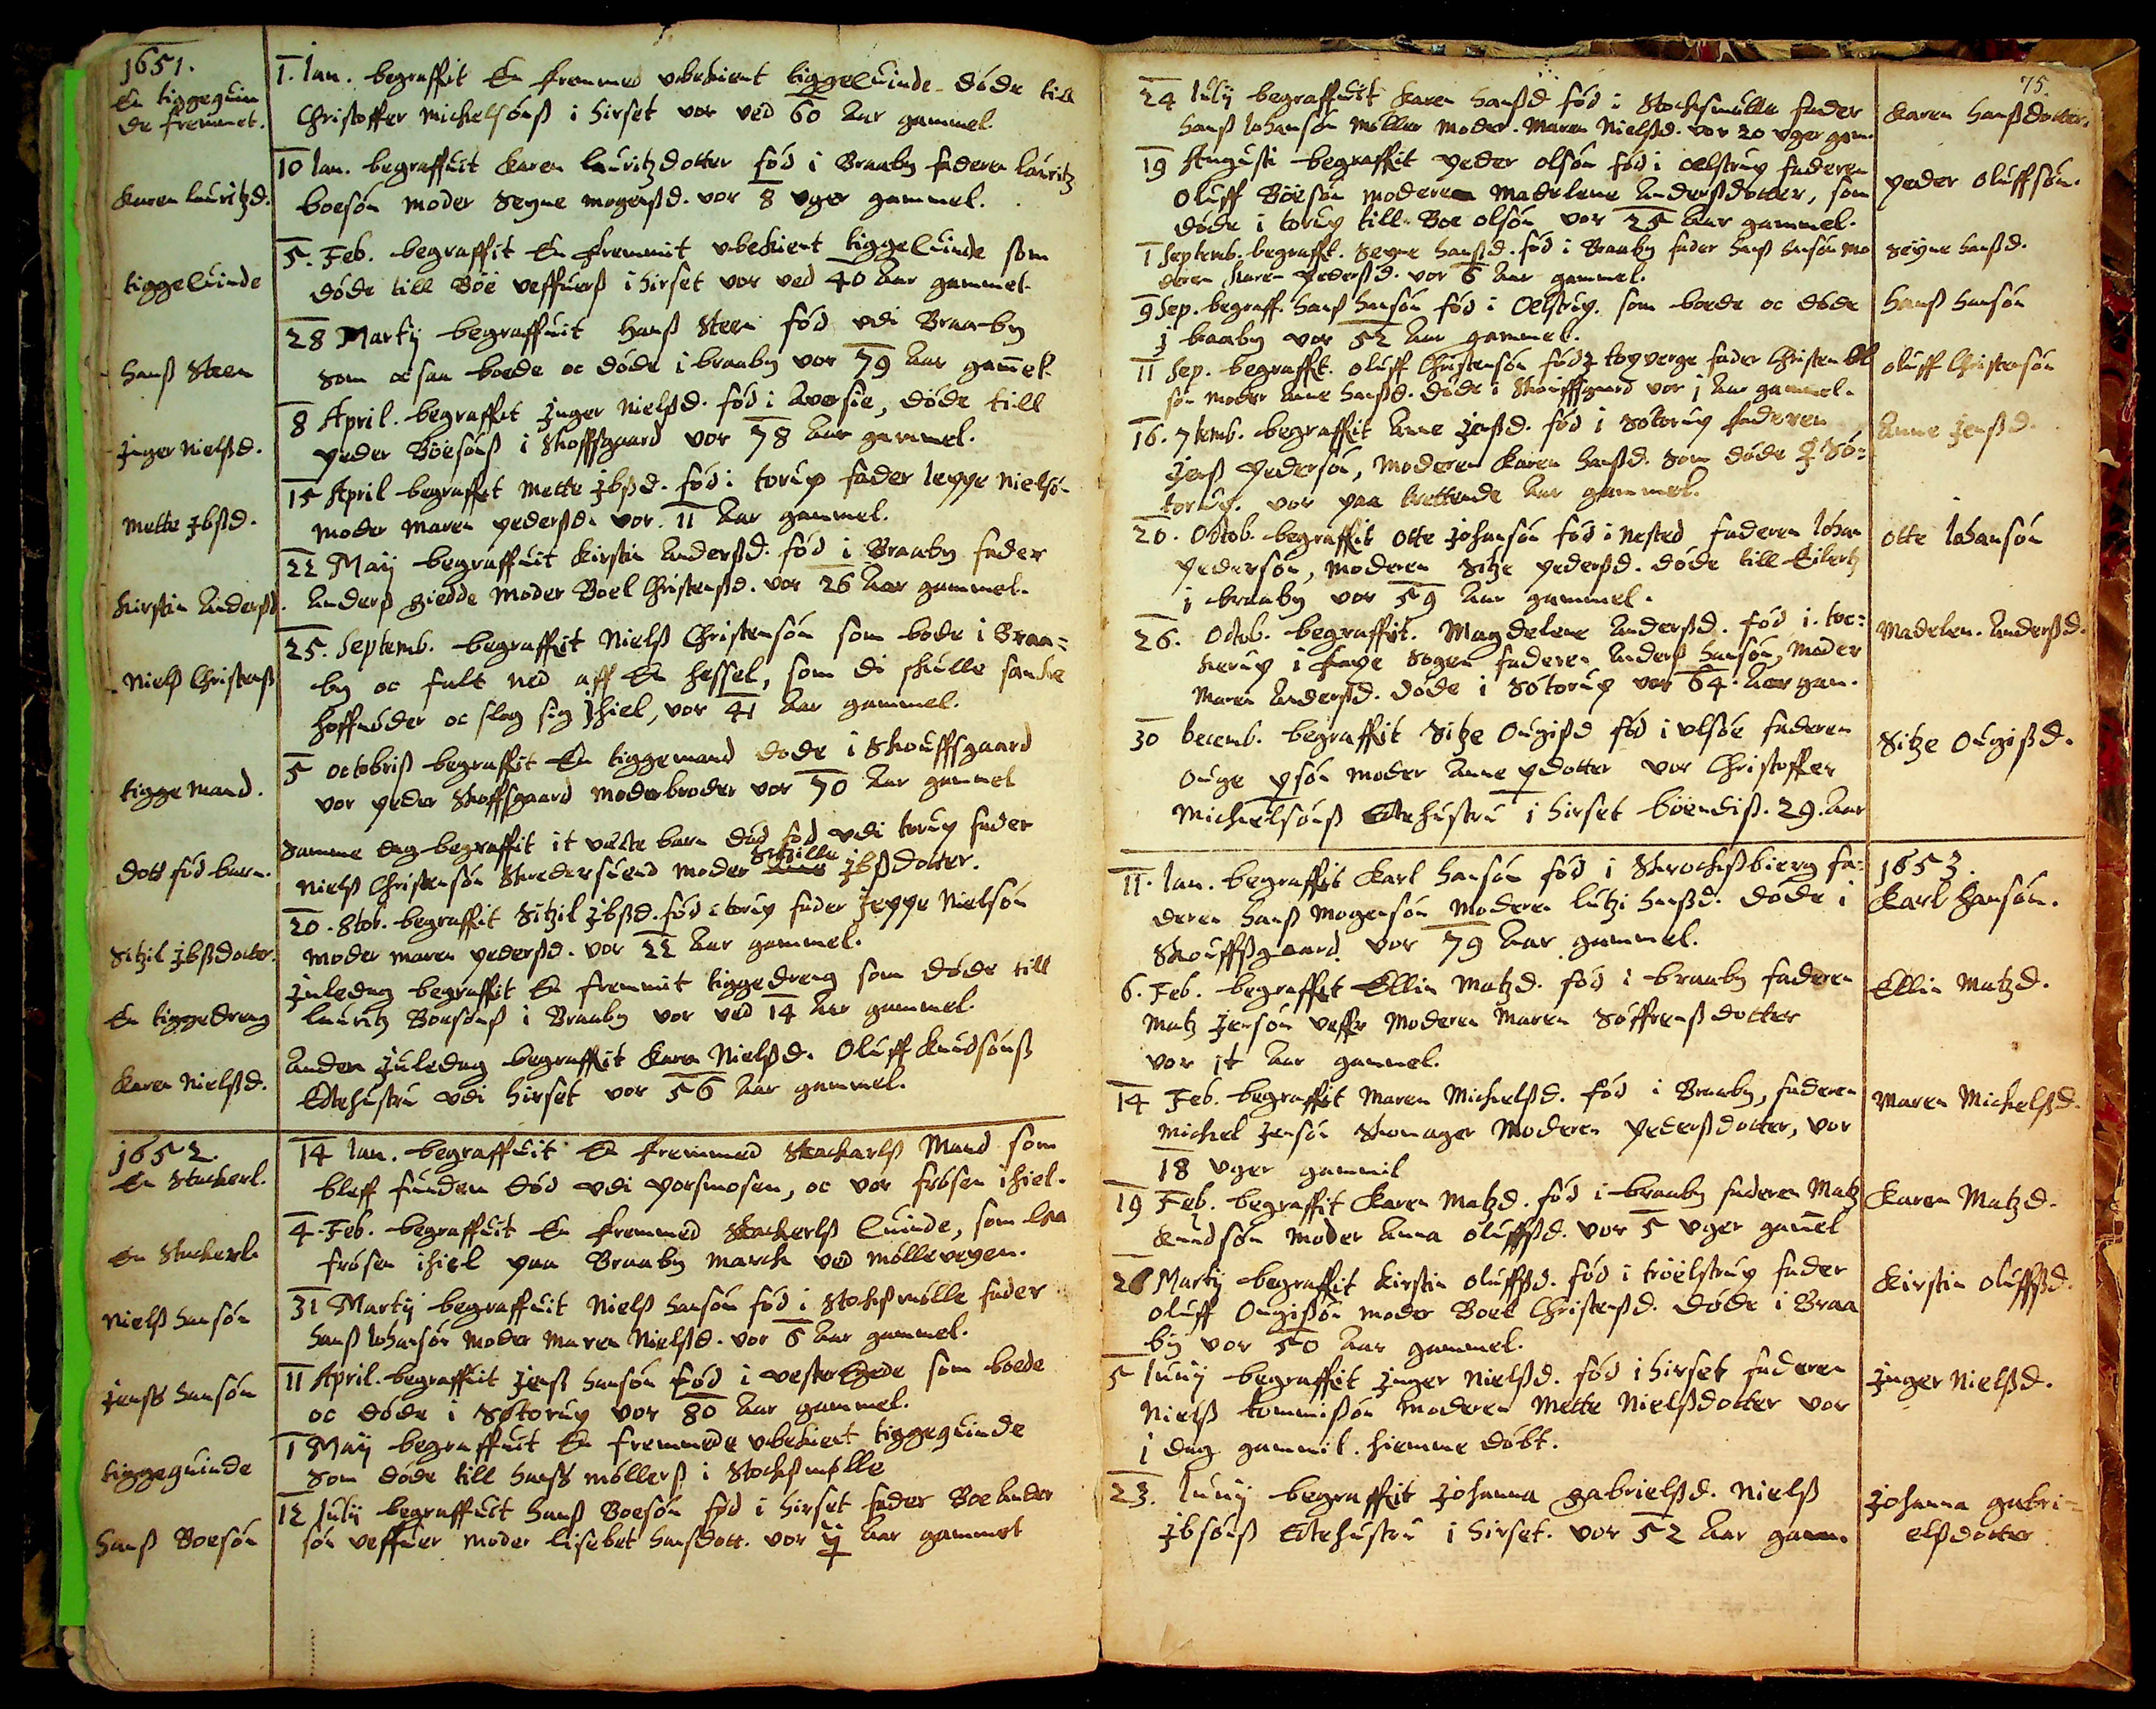1651.
En Tiggequin
de fremmet
1. Jan. begraffit En fremmed ubekient TiggeQuinde, døde till
Christoffer Michelsøns i Hirset var ved 60 Aar gammel. 
Karen Lauritzd. 
10 Jan. begraffuit Karen Lauritzdotter fød i Braaby Faderen Lauritz
Boesøn Moder Segne Mogensd. var 8 Uger gammel.
TiggeQuinde
5. Feb. begraffit En fremmit ubekient tiggeQuinde som
døde till Boe Veffuers i Hirset var ved 40 Aar gammel.
Hans Steen
28 Marty begraffuit Hans Steen fød udi Braaby
som ocsaa boede oc døde i Braaby, var 79 Aar gammel.
Jnger Nielsd.
8 April. begraffit Jnger Nielsd. fød i Aversie, døde till
Peder Boesøns i Skoffgaard var 78 Aar gammel.
Mette Jbsd. 
14. April begraffit Mette Jbsd fød i Torup Fader Jeppe Nielsøn
Moder Maren Pedersd. var 11 Aar gammel.
Kirstin Andersd.
22 May begraffuit Kirsten Andersd. fød i Braaby Fader
Anders Giedde Moder Boel Christensd. var 26 Aar gammel.
Niels Christensøn
25. Septemb. begraffuit Niels Christensøn som boede i Braa¬
by oc falt ned aff en Hassel, som di skulle samle
Hassnøder oc slog sig Ihiel, var 41 Aar gammel.
Tiggemand
5. Octobris begraffit En tiggemand døde i Skouffsgaard
var Peder Skoffsgaard Moderbroder var 70 Aar gammel.
Død fød barn
Samme dag begraffit it uæchte barn dødfød udi Torup Fader
Sitzille
Niels Christensøn Skredersuend Moder ## Jbsdatter.
Sitzil Jbsdatter.
20. 8tob. begraffit Sitzil Jbsd. fød i Torup Fader Jeppe Nielsøn
Moder Maren Pedersd. var 22 Aar gammel.
En Tiggedreng
Juledag begraffit En fremmit Tiggedreng som døde till
Lauritz Boesøns i Braaby var ved 14 Aar gammel.
Karen Nielsd.
Anden Juledag begraffit Karen Nielsd. Oluf Knudsøns
Echtehustru udi Hirset var 56 Aar gammel
1652.
1652 En Stacharl.
14 Jan begraffuit En fremmed Stacharls Mand som
bleff funden død udi Porsmosen, oc var frøsen ihiel.
En Stacharl. 
4. Feb. begraffuit En fremmed Stacharls Quinde, som laa
frøsen ihiel paa Braaby March ved Møllevegen.
Niels Hansøn
31. Marty begraffuit Niels Hansøn fød i Stochsmølle Fader
Hans Johansøn Moder Maren Nielsd. var 6 Aar gammel.
Jens Hansøn
11 April begraffit Jens Hansøn fød i VesterEgede som boede
oc døde i Søtorup var 80 Aar gammel.
TiggeQuinde
1 May begraffuit En fremmed ubekient TiggeQuinde
som døde till Hans Møllers i Stochsmølle.
Hans Boesøn
12 July begraffuit Hans Boesøn fød i Hirset Fader Boe Ander
søn veffuer Moder Lisebet Hansdatt. var 1½  Aar gammel.
24 July begraffuit Karen Hansd. fød i Stochsmølle Fader
Hans Johansøn Møller Moder Maren Nielsd. var 20 Uger gammel.
75.
Karen Hansdatter.
19 Augusti begraffit Peder Olsøn fød i Oelstrup Faderen
Oluff Boesøn Moderen Madelene Andersdatter, som
døde i Torup till Boe Olsøn var 25 Aar gammel.
Peder Oluffsøn
1 Septemb. begraffit Segne Hansd. fød i Braaby Fader Hans Hansøn Mo
der Karen Pedersd. var 6 Aar gammel.
Segne Hansd.
9 Sep. begraff. Hans Hansøn fød i Oelstrup, som boede oc døde
J Braaby var 52 Aar gammel.
Hans Hansøn
11 Sep. begraffit Oluff Christensøn fød J Toxverge## Fader Christen Ol
søn Moder Anne Hansd. døde i Skouffsgaard var 1 Aar gammel.
Oluff Christensøn
16. 7temb. Begraffit. Ane Jensd. fød i Søtorup Faderen
Jens Pedersøn, Moderen Karen Hansd. som døde J Sø¬
torup, var på trettende Aar gammel.
Anne Jensd.
20. Octob. begraffit Otte Johansøn fød i Nestved Faderen Johan
Pedersøn, Moderen Sitze Pedersd. døde till Eilertz
i Braaby, var 59 Aar gammel.
Otte Johansøn
26. Octob. begraffit Magdelene Andersd. fød i Toc¬
herup i Faxe sogn Faderen Anders Hansøn, Moder
Maren Andersd. døde i Søtorup var 64. Aar gammel.
Madelene Andersd.
30 Decemb. begraffit Sitze Ougisd. fød i Ulsøe Faderen
Ouge P.søn Moder Anne P. dotter var Christoffer
Michelsøns Ectehustru i Hirset boendis. 29. Aar.
Sitze Ougisd.
11.Jan. begraffit Karl Hansøn fød i Skrochsbierg## Fa¬
deren Hans Mogensøn Moderen Lutzi Hansd. døde i
Skouffsgaard var 79 Aar gammel.
1653.
Karl Hansøn
6. Feb. begraffit Ellin Matzd. fød i Braaby Faderen
Matz Jensøn Veffer Moderen Maren Søffrensdotter 
var 1½ Aar gammel.
Ellin Matzd.
14 Febr. begraffit Maren Michelsd. fød i Braaby, Faderen
Michel Jensøn Skoemager Moder Pedersdatter, var
18 Uger gammel.
Maren Michelsd.
19 Feb. begraffit Karen Matzd. fød i Braaby Faderen Matz
Knudsøn, Moder Anna Oluffsd. var 5 Uger gammel.
Karen Matzd.
26 Marty begraffit Kirstin Oluffsd. fød i Troelstrup Fader
Oluf Ougisøn, Moder Boel Christensd. døde i Braa
by var 50 Aar gammel.
Kirstin Oluffsd.
5 Juny begraffit Jnger Nielsd. fød i Hirset Faderen
Niels Tommisøn Moderen Mette Nielsdatter var
1 dag gammel. hiemme døbt.
Jnger Nielsd.
23. Juny begraffuit Johanne Gabrielsd. Niels
Jbsøns Ectehustru i Hirset. var 52 Aar gammel.
Johanne Gabri¬
elsdatter